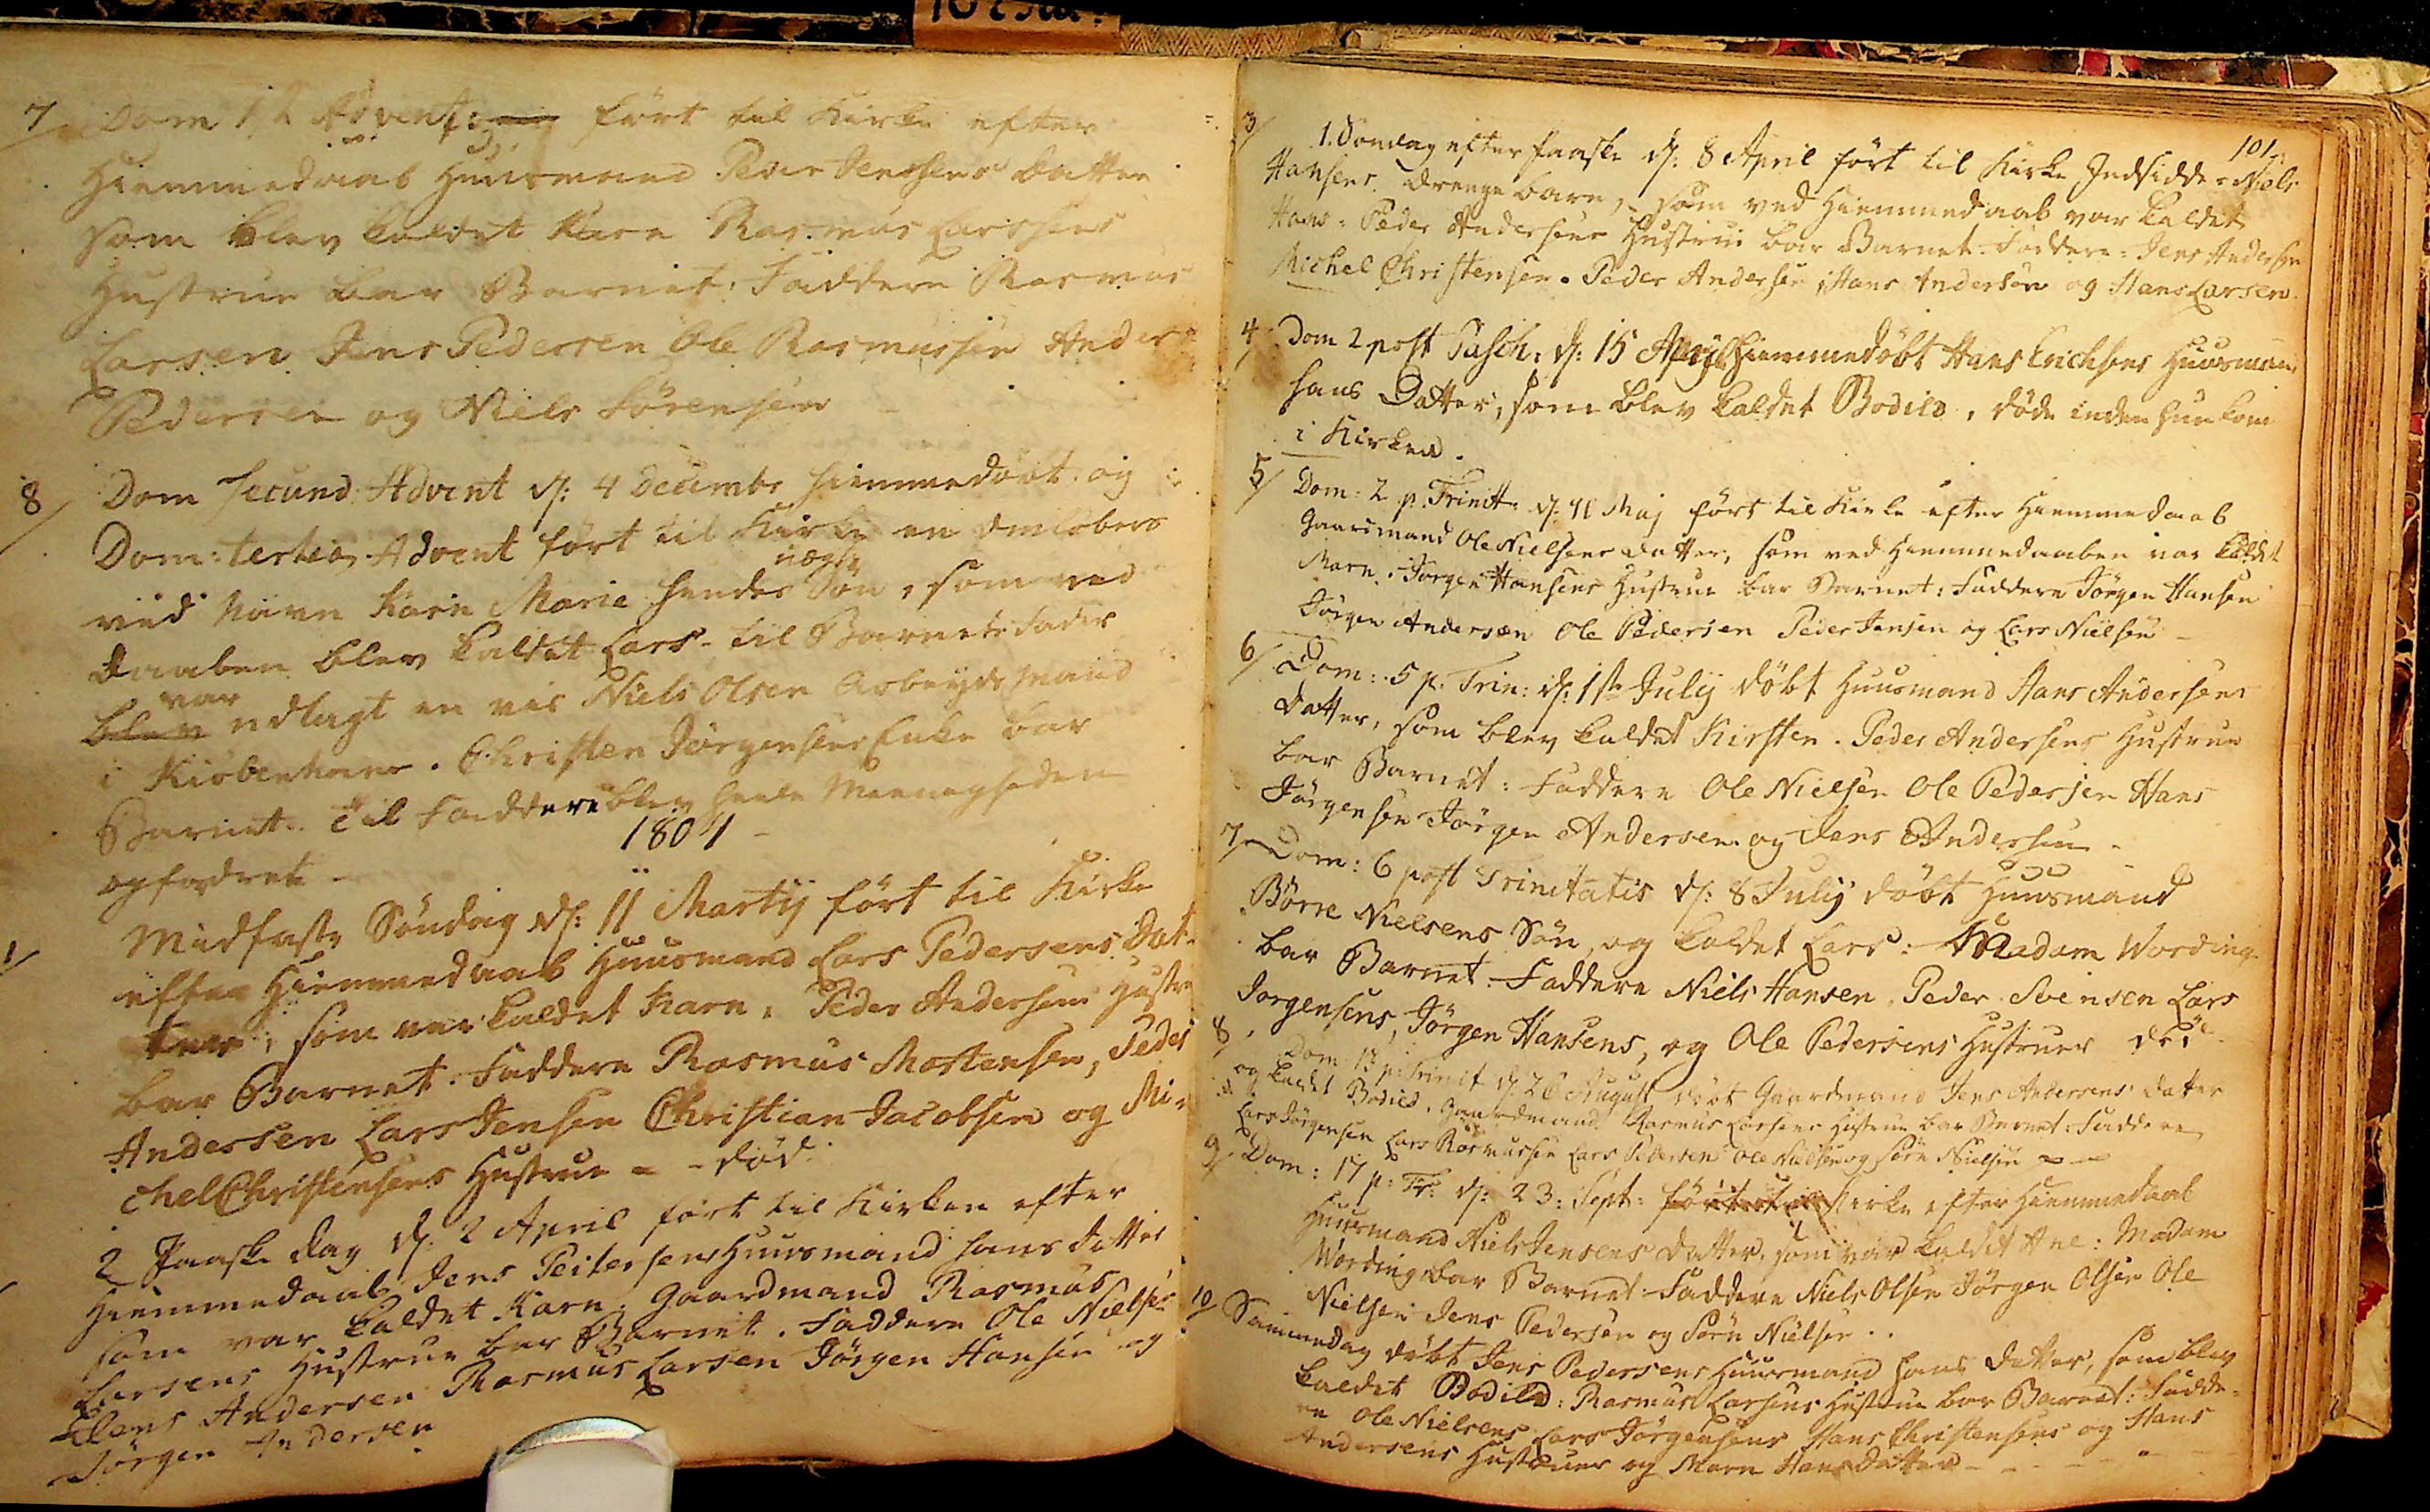7/ Dom 1ste Advent:       ført til Kirke efter
Hiemmedaab Huusmand Peder Jensens Datter
som blev kaldet Karn. Rasmus Larssens
Hustrue bar Barnet. Faddere Rasmus
Larsen Jens Pedersen Ole Rasmussen Anders
Pedersen og Niels Sørensen 
8/ Dom Secund: Advent d: 4 Decembr hiemmedøbt, og
Dom: Tertia Advent ført til Kirke en Omløbers
uægte
ved Navn Karn Marie hendes Søn, som ved
Daaben blev kaldet Lars. til barnets Fader
var
blev udlagt en vis Niels Olsen Arbeijds Mand
i Kiøbenhavn. Christen Jørgensens Enke bar
Barnet. Til Faddere blev heele Meenigheden
opfordret
1804 
1/ Midfaste Søndag d: 11 Martij ført til Kirke
efter Hiemmedaab Huusmand Lars Pedersens Dat¬
ter, som var kaldet Karn, Peder Andersens Hustru
bar Barnet. Faddere Rasmus Mortensen, Peder
Andersen Lars Jensen Christian Jacobsen og Mi¬
chel Christensens Hustrue - død.
2 Paaske Dag d: 2 April ført til Kirken efter
Hiemmedaab Jens Peitersens Huusmand hans Datter
som var kaldet Karn. Gaardmand Rasmus
Larsens Hustrue bar Barnet. Faddere Ole Nielsen
Claus Andersen Rasmus Larsen Jørgen Hansen og
Jørgen Andersen
101.
3/ 1. Søndag efter Paaske d: 8 April ført til Kirke Indsidder Niels
Hansens Drengebarn, som ved Hiemmedaab var kaldet
Hans: Peder Andersens Hustrue bar Barnet. Faddere: Jens Andersen
Michel Christensen. Peder Andersen; Hans Andersen og Hans Larsen.
4/ Dom 2 post Pasch: d: 15 April hiemmedøbt Hans Erichsens Huusmand
hans Datter, som blev kaldet Bodild, døde inden hun kom
i Kirken.
5/ Dom: 2. p: Trinit: d: 10 Maj ført til Kirke efter Hiemmedaab
Gaardmand Ole Nielsens Datter, som ved Hiemmedaaben var kaldt
Marn. Jørgen Hansens Hustrue bar Barnet: Faddere Jørgen Hansen
Jørgen Andersen Ole Pedersen Peder Jensen og Lars Nielsen
6/ Dom: 5 p: Trin: d: 1ste Julij døbt Huusmand Hans Andersens
Datter, som blev kaldet Kirsten. Peder Andersens Hustrue
bar Barnet: Faddere Ole Nielsen Ole Pedersen Hans
Jørgensen Jørgen Andersen og Jens Andersen.
7/ Dom: 6 post Trinitatis d: 8 Julij døbt Huusmand
Børre Nielsens Søn og kaldet Lars: Madam Wording
bar Barnet: Faddere Niels Hansen, Peder Svensen Lars
Jorgensens, Jørgen Hansens, og Ole Pedersens Hustruer død
8/ Dom: 13 p: Trinit d: 26 August døbt Gaardmand Jens Andersens datter
og kaldt Bodild. Gaardmand Rasmus Larsens Hustrue bar Barnet: Faddere
Lars Jørgensen Lars Rasmussen Lars Pedersen Ole Nielsen og Sørn Nielsen
9/ Dom: 17 p: Tr: d: 23: Sept: ## ført til Kirke efter Hiemmedaab
Huusmand Niels Jensens Datter, som var kaldet Ane: Madam
Wording bar Barnet. Faddere Niels Olsen Jørgen Olsen Ole
Nielsen Jens Pedersen og Sørn Nielsen.
10/ Sammedag døbt Jens Pedersens Huusmand hans Datter, som blev
kaldet Bodild: Rasmus Larsens Hustrue bar Barnet: Fadde¬
re Ole Nielsens Lars Jørgensens Hans Christensens og Hans
Andersens Hustruer og Marn Hans Datter
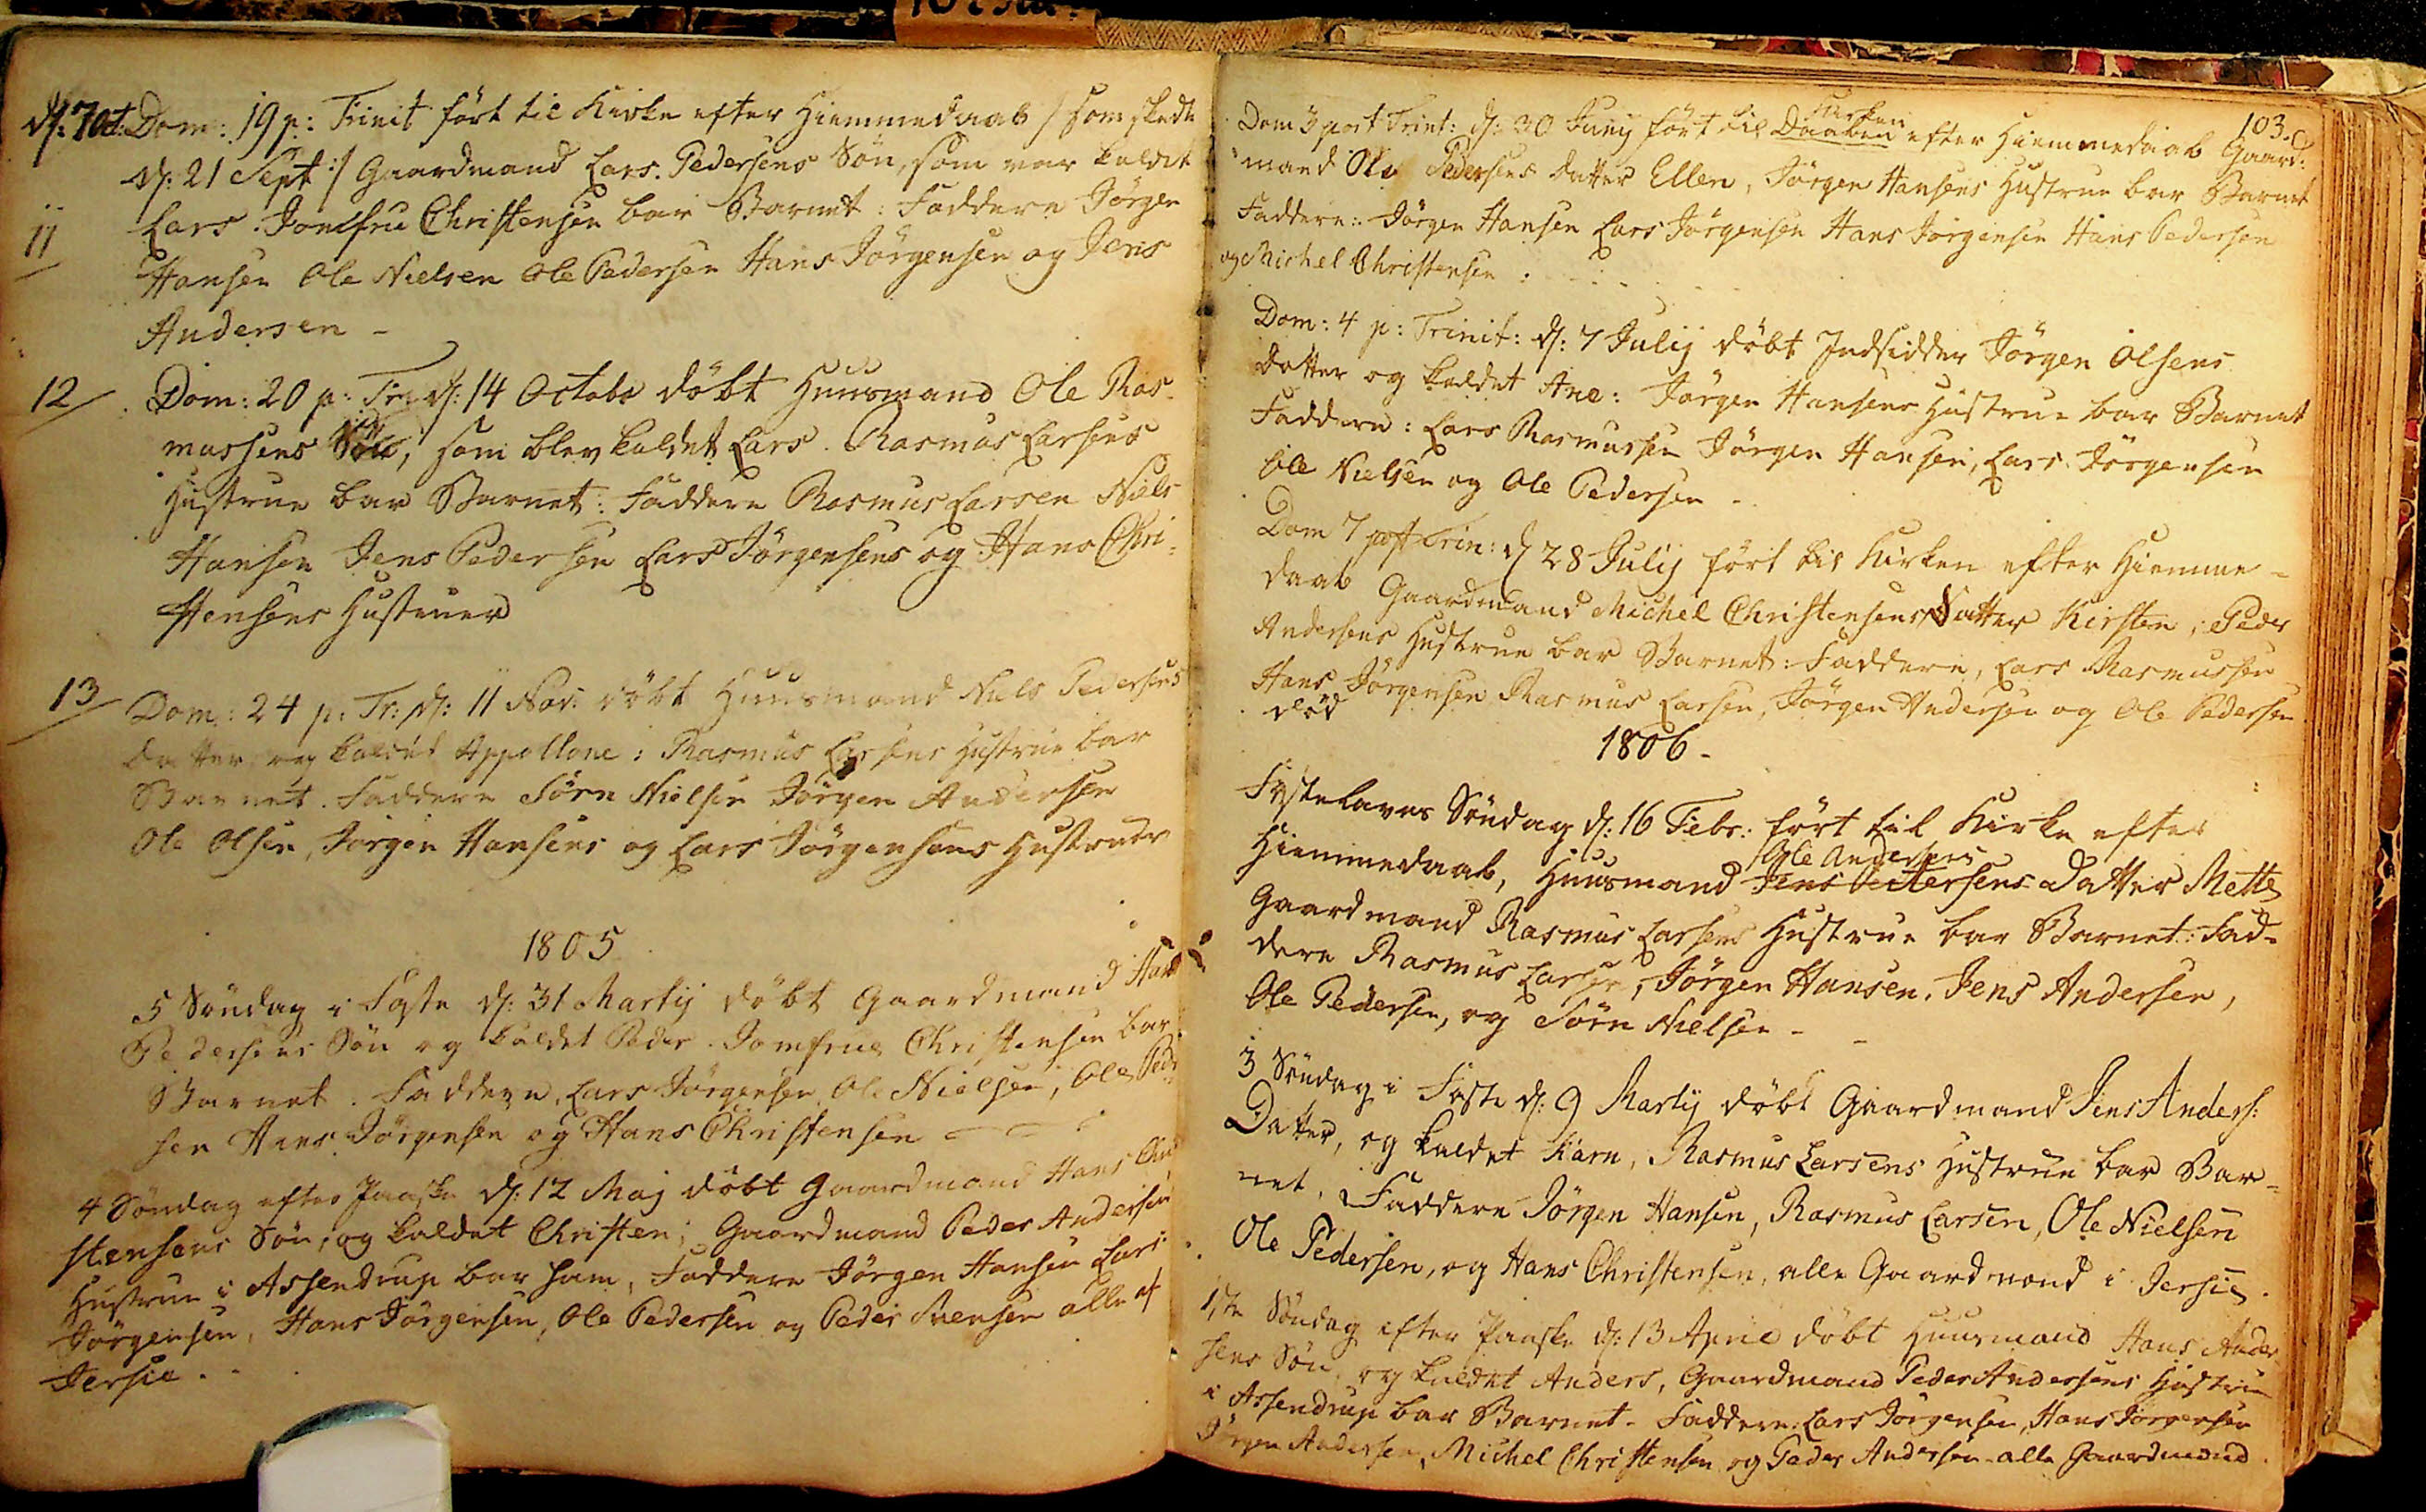d: 7 oct: Dom: 19 p: Trinit ført til Kirke efter Hiemmedaab / Som skedte
d: 21 Sept: / Gaardmand Lars Pedersens Søn, som var kaldet
11/ Lars. Jomfru Christensen bar Barnet: Faddere Jørgen
Hansen Ole Nielsen Ole Pedersen Hans Jørgensen og Jens
Andersen
12/ Dom: 20: Tr: d: 14 Octobr døbt Huusmand Ole Ras
mussens Søn, som blev kaldet Lars. Rasmus Larsens
Hustrue bar Barnet: Faddere Rasmus Larsen Niels
Hansen Jens Pedersen Lars Jørgensens og Hans Chri¬
stensens Hustruer
13/ Dom: 24 p: Tr: d: 11 Nov: døbt Huusmand Niels Pedersens
Datter, og kaldet Appollone: Rasmus Larsens Hustrue bar
Barnet. Faddere Sørn Nielsen Jørgen Andersen
Ole Olsen, Jørgen Hansens og Lars Jørgensens Hustruer
1805
5 Søndag i Faste d: 31 Martij døbt Gaardmand Hans
Pedersens Søn og kaldet Peder: Jomfrue Christensen bar
Barnet: Faddere, Lars Jørgensen, Ole Nielsen, Ole Peder
sen Hans Jørgensen og Hans Christensen
4 Søndag efter Paaske d: 12 Maj døbt Gaardmand Hans Chri
stensens Søn, og kaldet Christen; Gaardmand Peder Andersens
Hustrue i Assendrup bar ham, Faddere Jørgen Hansen Lars
Jørgensen, Hans Jørgensen, Ole Pedersen og Peder Svensen alle af
Jersie
103.
Kirken
Dom 3 post Trinit: d: 30 Junij ført til Daaben efter Hiemmedaab Gaard:
mand Ole Pedersens Datter Ellen, Jørgen Hansens Hustrue bar Barnet
Faddere: Jørgen Hansen Lars Jørgensen Hans Jørgensen Hans Pedersen
og Michel Christensen: .....

Dom: 4 p: Trinit: d: 7 Julij døbt Indsidder Jørgen Olsens
Datter og kaldet Ane: Jørgen Hansens Hustrue bar Barnet
Faddere: Lars Rasmussen Jørgen Hansen Lars Jørgensen
Ole Nielsen og Ole Pedersen
Dom 7 post Trin: d 28 Julij ført til Kirken efter Hiemme¬ 
daab Gaardmand Michel Christensens Datter Kirsten; Peder
Andersens Hustrue bar Barnet: Faddere, Lars Rasmussen
Hans Jørgensen, Rasmus Larsen, Jørgen Andersen og Ole Pedersen
død
1806
Fastelavns Søndag d: 16 Febr: ført til Kirke efter
Ole Andersens
Hiemmedaab, Huusmand Jens Peitersens Datter Mette
Gaardmand Rasmus Larsens Hustrue bar Barnet: Fad¬
dere Rasmus Larsen, Jørgen Hansen, Jens Andersen,
Ole Pedersen, og Sørn Nielsen - -
3 Søndag i Faste d: 9 Martij døbt Gaardmand Jens Anders:
Datter, og kaldet Karn, Rasmus Larsens Hustrue bar Bar¬
net, Faddere Jørgen Hansen, Rasmus Larsen, Ole Nielsen
Ole Pedersen, og Hans Christensen, alle Gaardmænd i Jersie.
1ste Søndag efter Paaske d: 13 April døbt Huusmand Hans Ander
sens Søn, og kaldet Anders, Gaardmand Peder Andersens Hustrue
i Assendrup bar Barnet. Faddere: Lars Jørgensen, Hans Jørgensen
Jørgen Andersen, Michel Christensen, og Peder Andersen. alle Gaardmænd.